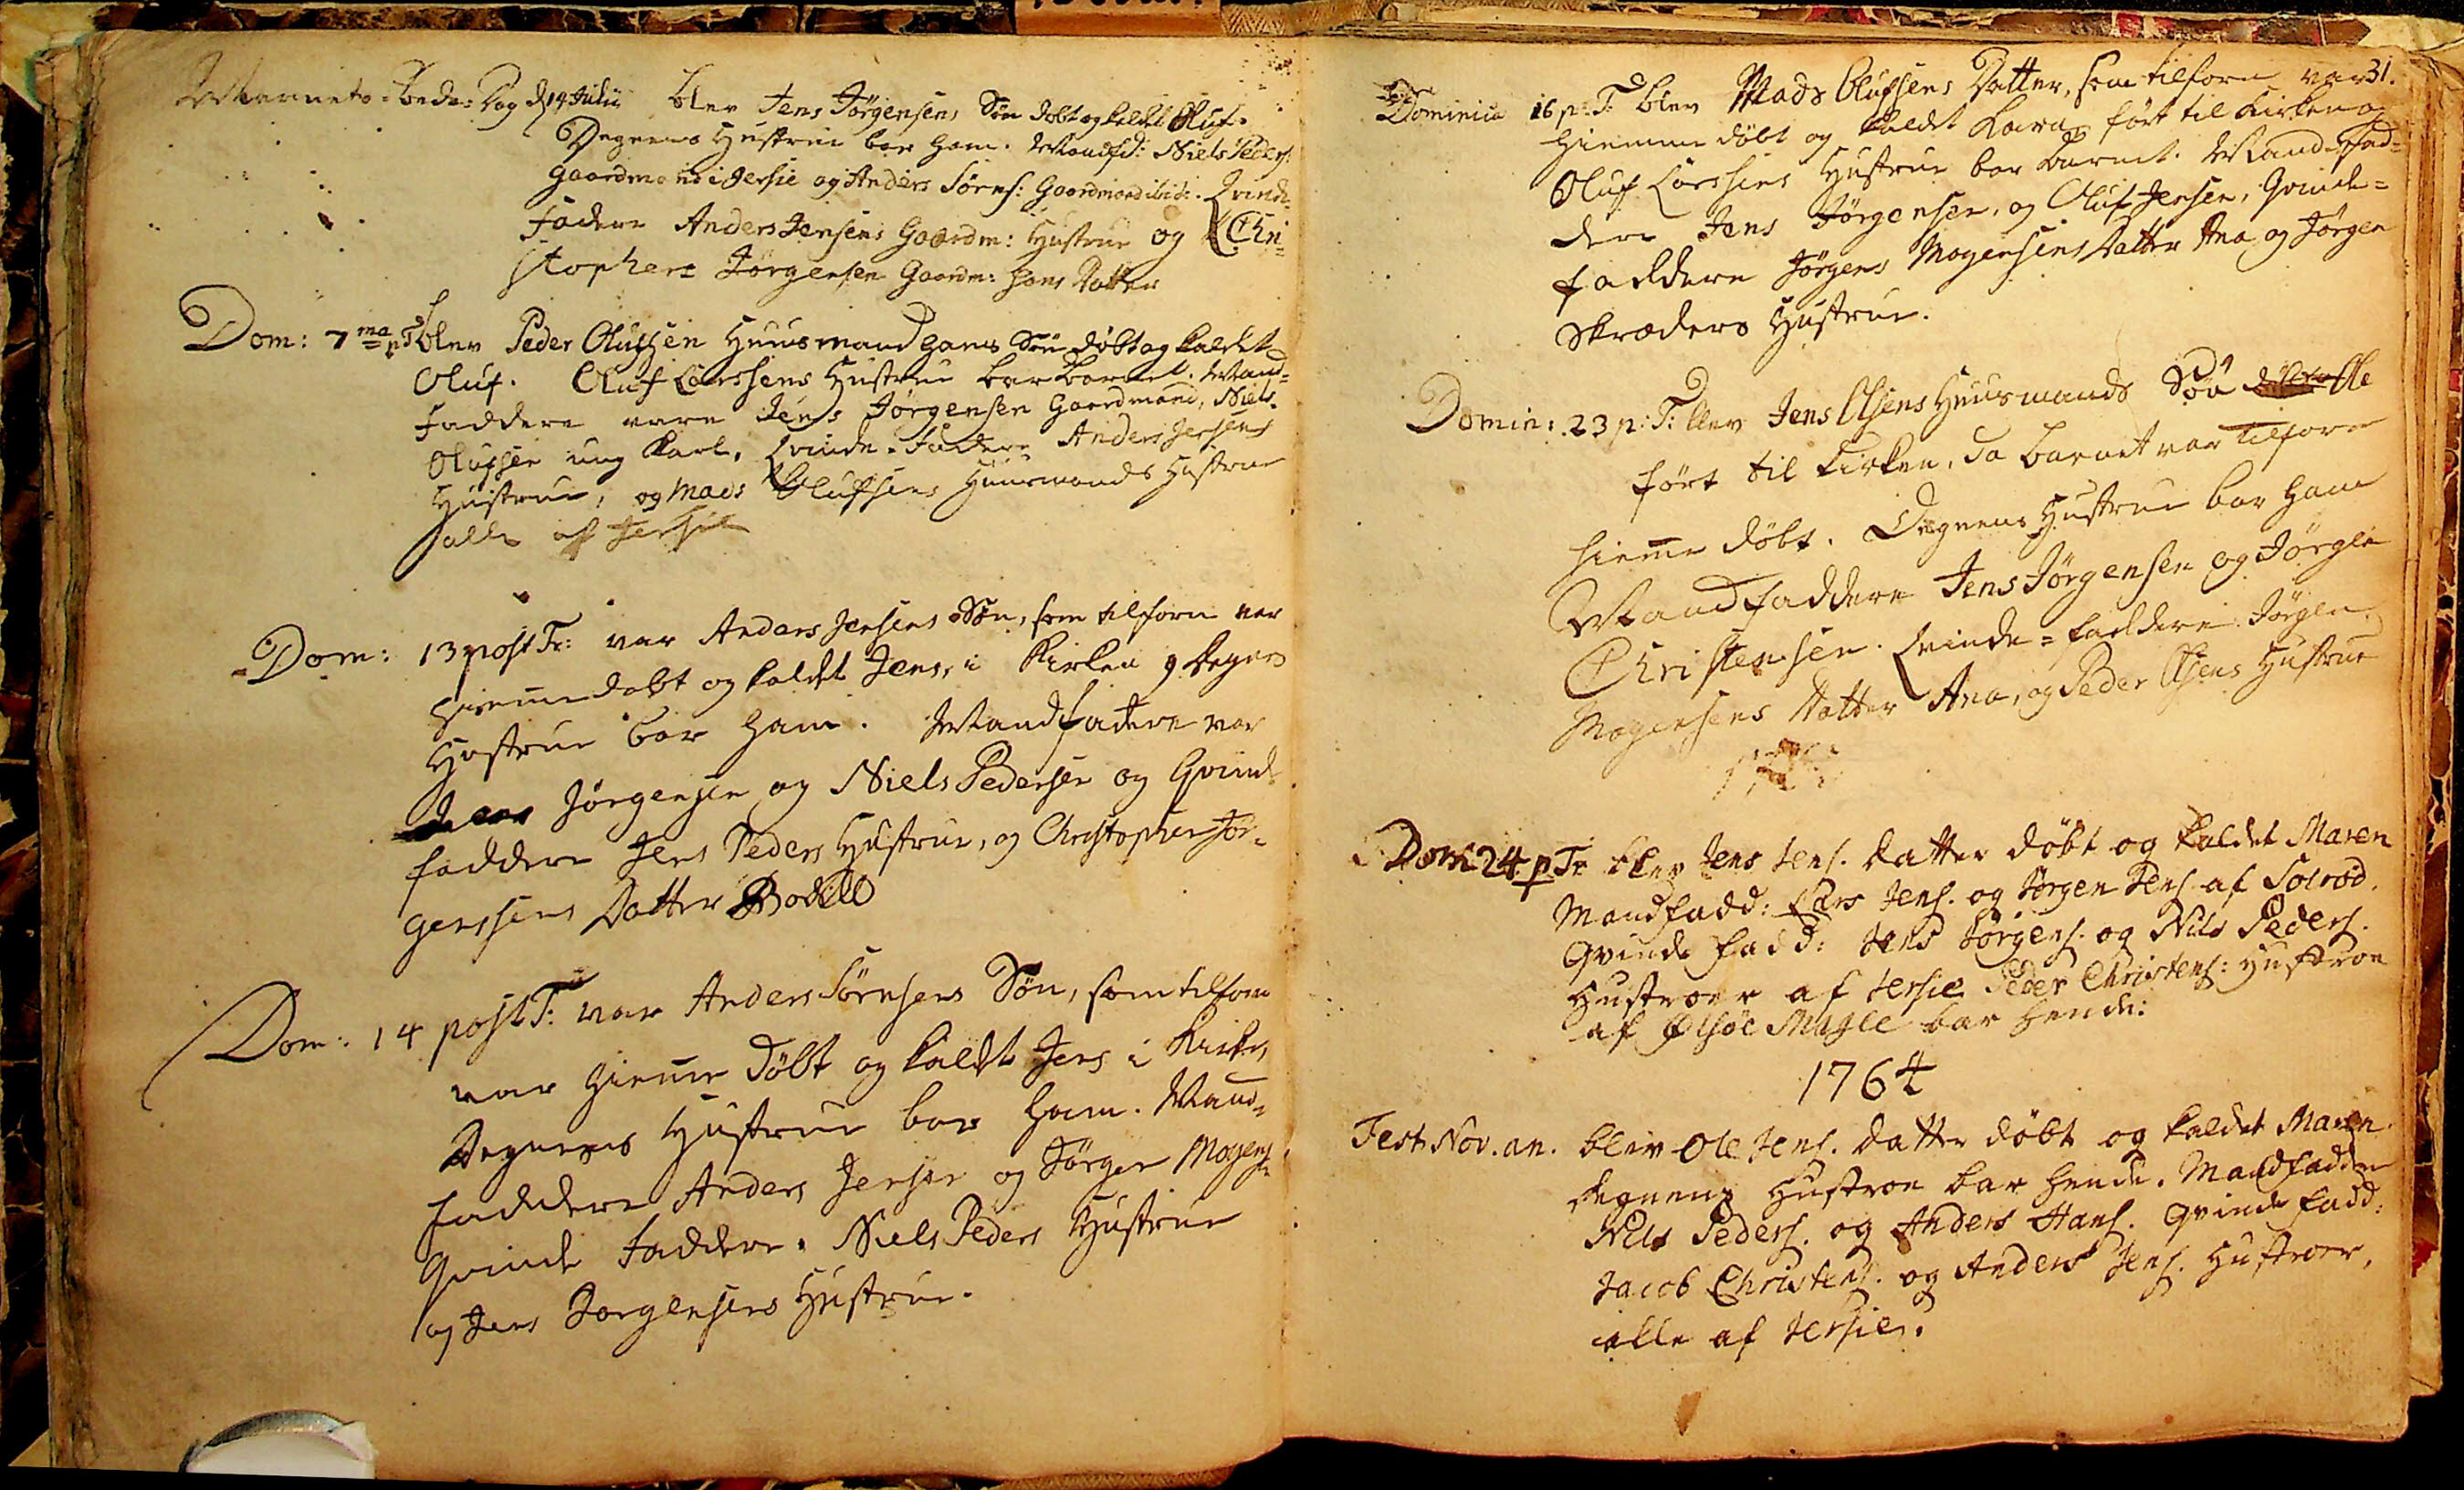Maanets-Bede-Dag d 14 Julii Blev Jens Jørgensens Søn døbt og kaldet Oluf.
Degnens Hustrue bar ham. Mandfd.: Niels Peders:
Gaardmand i Jersie og Anders Sørens: Gaardmand ibid: Qvinde¬
Fadere Anders Jensens Gaardm: Hustrue og Chri¬
stopher Jørgensen Gaardm: hans Datter
Dom: 7ma p T Blev Peder Olufsen Huusmand Hans Søn døbt og kaldet
Oluf. Oluf Larsens Hustrue bar Barnet. Mand¬
Faddere vare Jens Jørgensen Gaardmand, Niels
Olufsen ung Karl. Qvinde-Faddere Anders Jensens
Hustrue, og Mads Olufsens Huusmands Hustrue
alle af Jersie 
Dom: 13 post Tr: var Anders Jensens Søn, som tilforn var
Hiemmedøbt og kaldet Jens, i Kirken og Degnens
Hustrue bar ham. Mandfadere var
Jens Jørgensen og Niels Pedersen og Qvinde
faddere Jens Peders Hustrue, og Christopher Jør¬
gensens Datter Bodell
Dom: 14 post T: var Anders Sørnsens Søn, som tilforn
var Hiemme døbt og kaldt Jens i Kirke, 
Degnens Hustrue bar ham. Mand¬
faddere Anders Jensen og Jørgen Mogensen
Qvinde Faddere. Niels Peders Hustrue
Jens Jørgensens Hustrue.
31.
Dominica 16 p: T: blev Mads Olufsens Datter, som tilforn var
Hiemme døbt og kaldt Lara ført til Kirken og
Oluf Larsens Hustrue bar Barnet. Mand fad¬
dere Jens Jørgensen, og Oluf Jensen, Qvinde¬
faddere Jørgen Mogensens Datter Ana og Jørgen
Skræders Hustrue.
Domin: 23 p: T: blev Jens Olsens Huusmands Søn døbt Ole
ført til Kirken, da Barnet var tilforn
hiemme døbt, Degnens Hustrue bar ham
Mandfaddere Jens Jørgensen og Jørgen
Christensen. Qvinde=faddere Jørgen 
Mogensens Datter Ana, og Peder Olsens Hustrue
Dom: 24 p Tr: blev Jens Jens. Datter døbt og kaldet Maren
Mandfadd: Lars Jens. og Jørgen Jens. af Solrød.
Qvinde fadd: Jens Jørgens. og Nils Peders.
Hustroer af Jersie Peder Christens. Hustroe
af Ølsøe Magle bar hende:
1764
Fest Nov. an. blev Ole Jens. Datter døbt og kaldet Maren
Degnens Hustroe bar hende. Mandfaddere
Nils Peders. og Anders Hans. og Qvinde fadd:
Jacob Christens. og Anders Jens. Hustroer.
alle af Jersie.
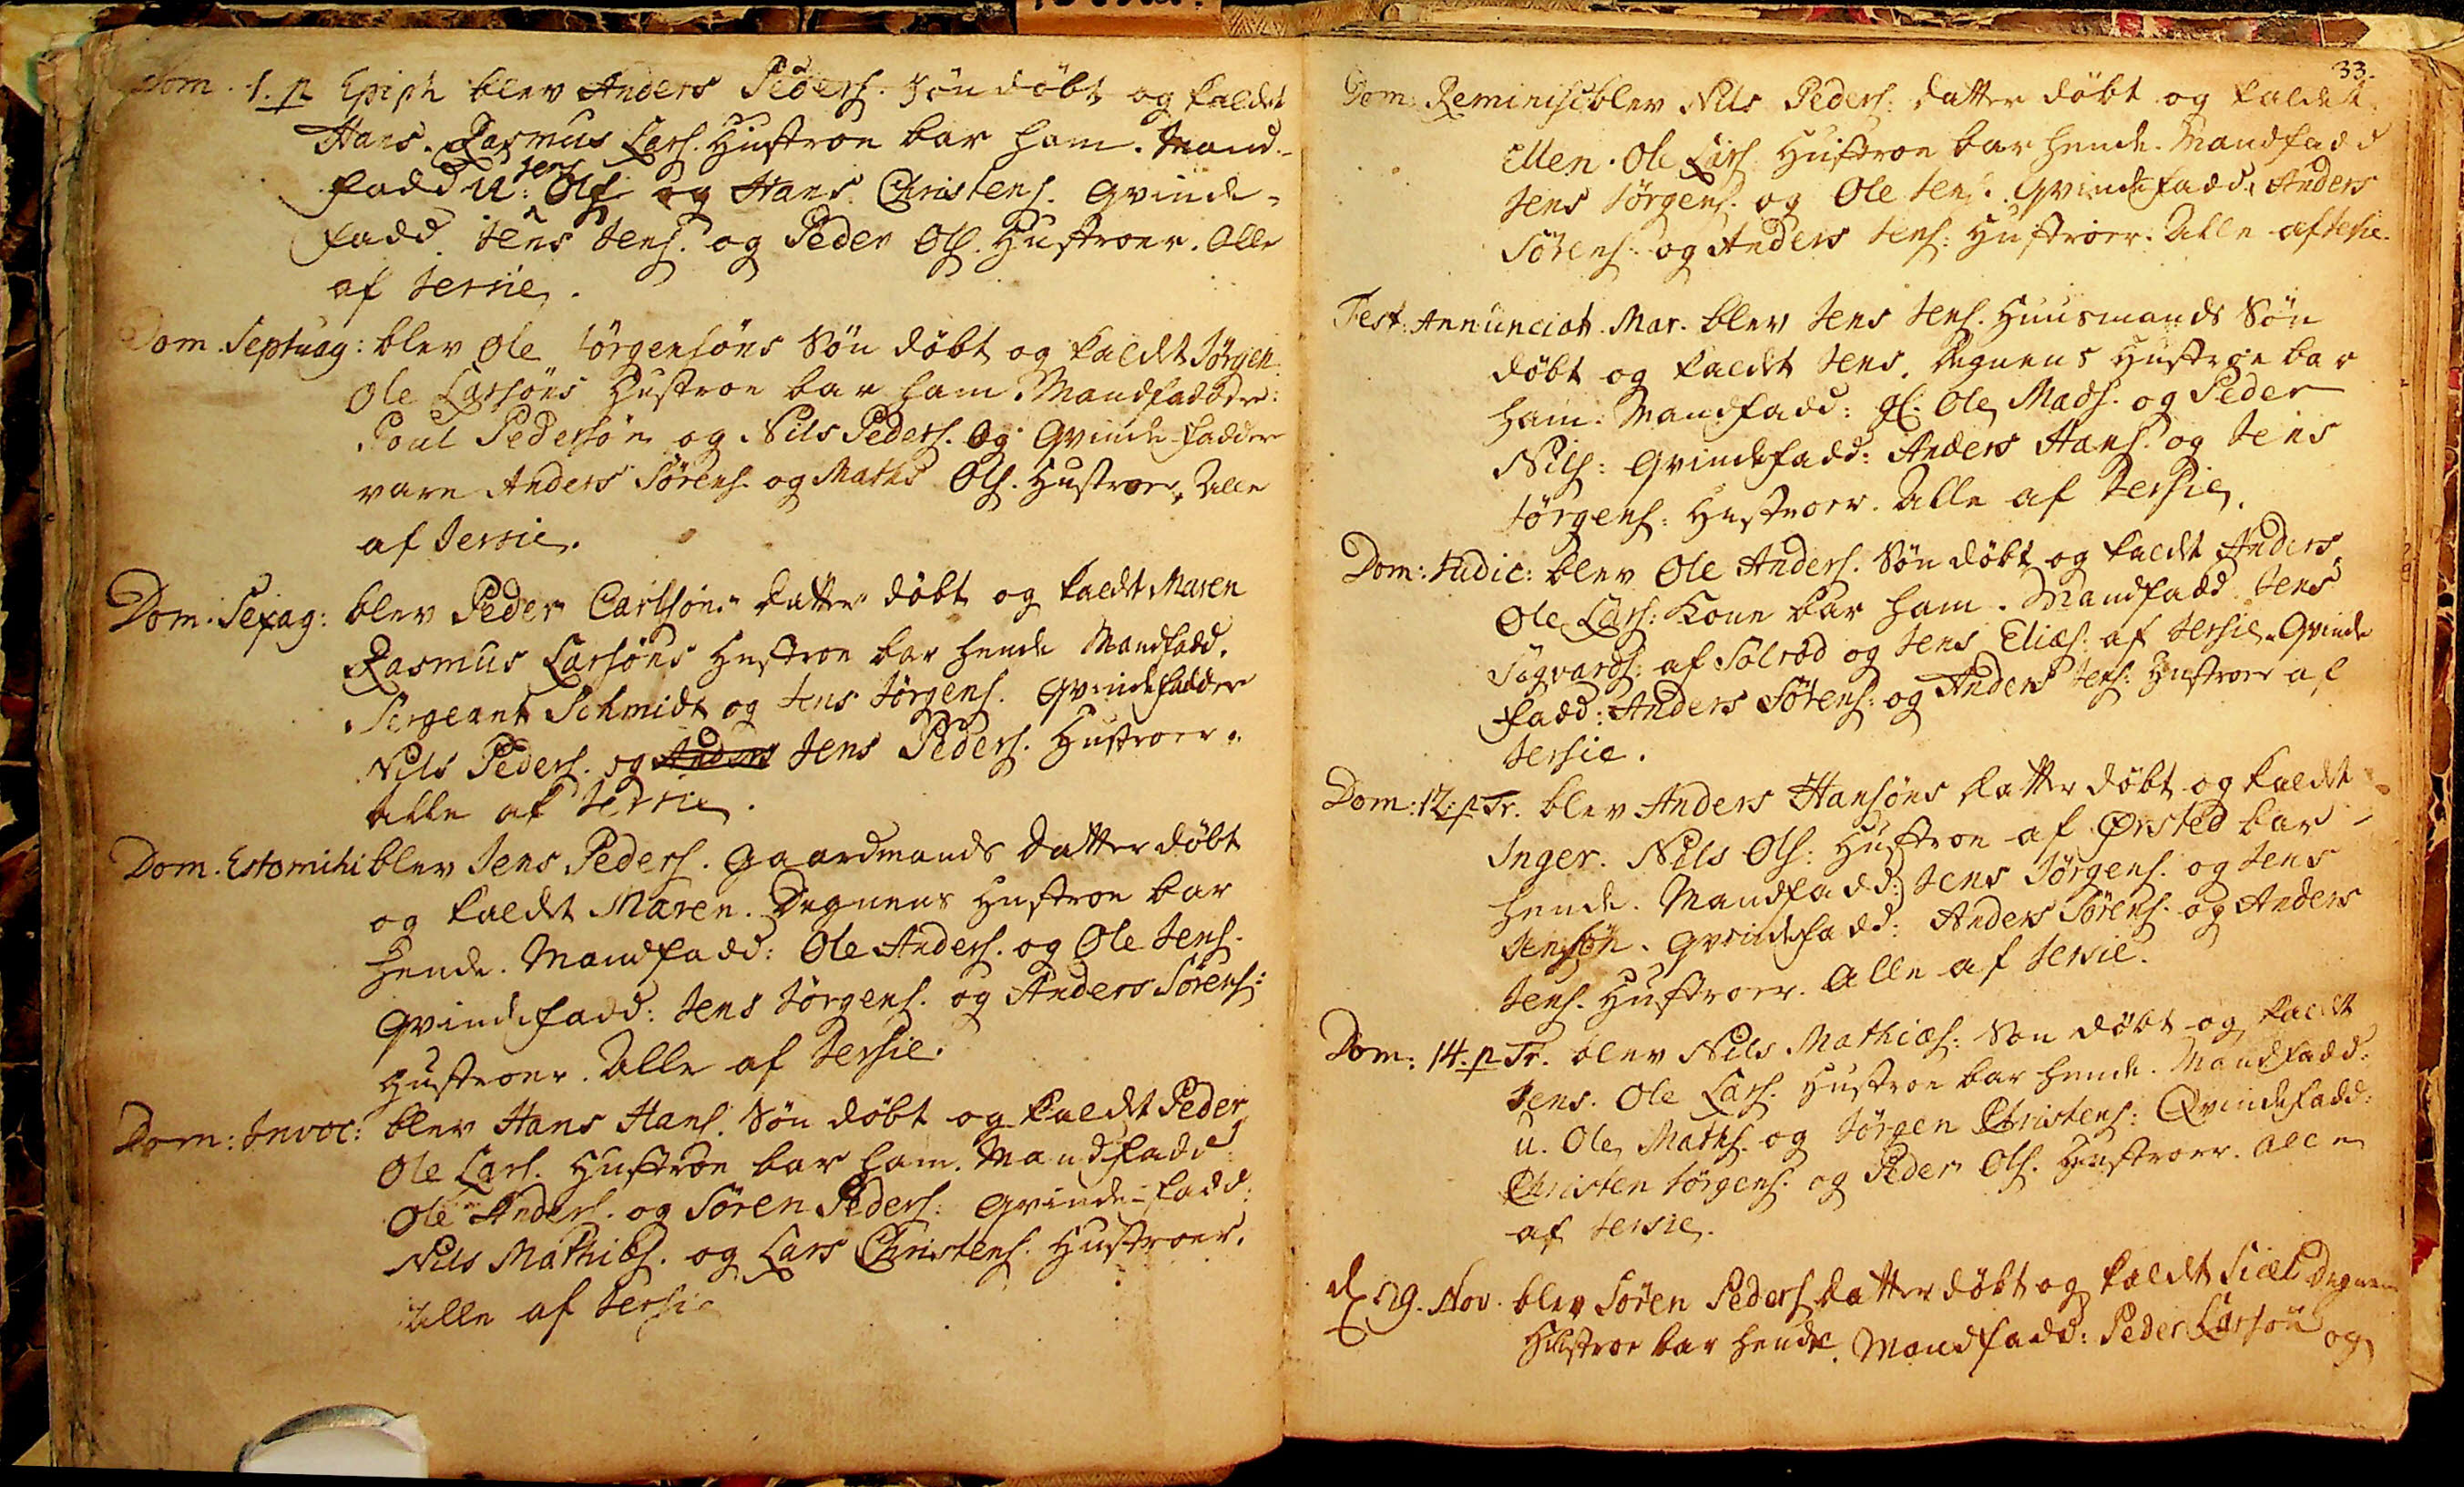Dom.1.p Epiph blev Anders Peders. Søn døbt og kaldet
Hans. Rasmus Lars. Hustroe bar ham. Mand¬
Jens
fadd U: Ols og Hans Christens. Qvinde¬
fadd. Jens Jens. og Peder Ols. Hustroer. Alle
af Jersie.
Dom. Septuag: blev Ole Jørgensøns Søn døbt og kaldt Jørgen
Ole Larsøns Hustroe bar ham. Mandfaddere:
Poul Pedersøn og Nils Peders. Og Qvinde-faddere
vare Anders Sørens. og Maths Ols. Hustroe. Alle
af Jersie.
Dom. Sexag: blev Peder Carlsøns Datter døbt og kaldt Maren
Rasmus Larsøns Hustroe bar hende Mandfadd.
Sergeant Schmidt og Jens Jørgens. Qvindefadder
Nils Peders. og Anders Jens Peders. Hustroer.
alle af Jersie
Dom. Estomihi blev Jens Peders. Gaardmands Datter døbt
og kaldt Maren. Degnens Hustroe bar
hende. Mandfadd: Ole Anders. og Ole Jens.
Qvindefadd: Jens Jørgens. og Anders Sørens:
Hustroer. Alle af Jersie.
Dom: Invoc: blev Hans Hans. Søn døbt og kaldt Peder
Ole Lars. Hustroe bar ham. Mandfadd:
Ole Anders. og Søren Peders: Qvinde-fadd:
Nils Mathiæs. og Lars Christens. Hustroer.
Alle af Jersie
33.
Dom. Reminisc: blev Nils Peders: Datter døbt og kaldet
Ellen. Ole Lars: Hustroe bar hende. Mandfadd
Jens Jørgens. og Ole Jens. Qvindefadd: Anders
Sørens: og Anders Jens: Hustroer. Alle af Jersie
Fest: Annunciat. Mar. blev Jens Jens. Huusmands Søn
døbt og kaldt Jens. Degnens Hustroe bar
ham. Mandfadd: gl. Ole Mads. og Peder
Nils: Qvindefadd: Anders Hans. og Jens
Jørgens: Hustroer. Alle af Jersie.
Dom: Judic: blev Ole Anders. Søn døbt og kaldt Anders
Ole Lars: Kone bar ham. Mandfadd: Jens
Sigvards: af Solrød og Jens Eliæs: af Jersie. Qvinde
fadd: Anders Sørens: og Anders Jens: Hustroer af
Jersie.
Dom: 12: p Tr. blev Anders Hansøns Datter døbt og kaldt
Inger. Nils Ols: Hustroe af Ørsted bar
hende. Mandfadd: Jens Jørgens. og Jens
Jensøn. Qvindefadd: Anders Sørens. og Anders
Jens. Hustroer. Alle af Jersie.
Dom: 14. p Tr. blev Nils Mathiæs: Søn døbt og kaldt
Jens. Ole Lars. Hustroe bar hende. Mand.fadd:
U. Ole Maths. og Jørgen Christens: Qvindefadd:
Christen Jørgens. og Peder Ols. Hustroer. Alle
af Jersie.
d 29. Nov.blev Søren Peders Datter døbt og kaldt Sicel Degnens
Hustroe bar hende. Mandfadd: Peder Larsøn og